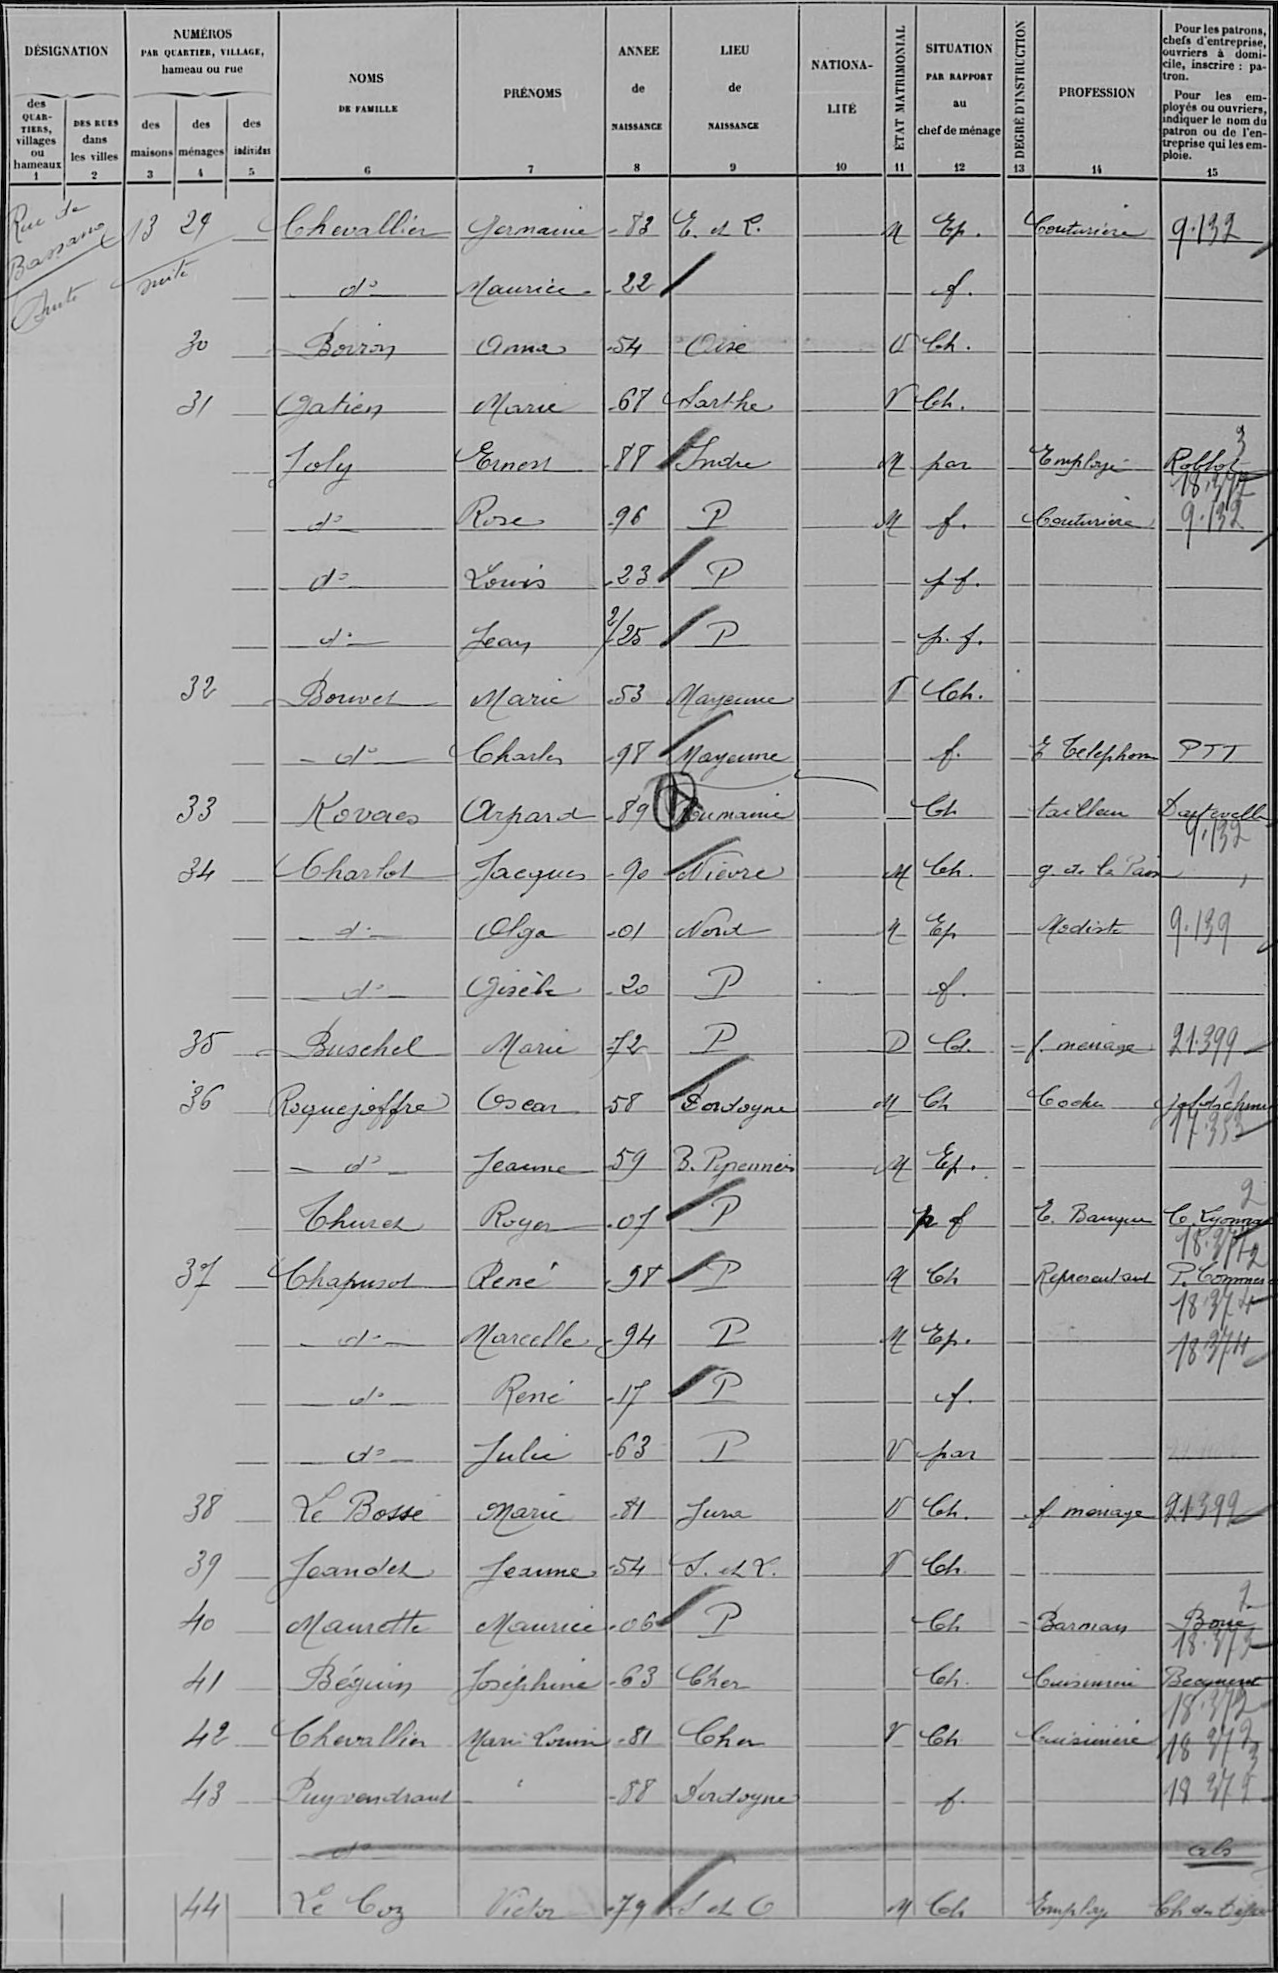Chevallier/Germaine/83/E et L /¤/M/Ep /¤/Couturiere/9132
d°/Maurice/22/¤/¤/¤/f/¤/¤/¤
Boiron/Annie/54/Oise/¤/V/Ch /¤/¤/¤
Gatien/Marie/67/Sarthe/¤/V/Ch /¤/¤/¤
Joly/Ernest/88/Indre/¤/M/par/¤/Employé/Roblot!18377
d°/Rose/96/P/¤/M/f /¤/couturiere/9132
d°/Louis/23/P/¤/¤/f f /¤/¤/¤
d°/Jean/25/P/¤/¤/f f /¤/¤/¤
Bouver/Marie/53/Mayenne/¤/V/Ch /¤/¤/¤
d°/Charles/98/Mayenne/¤/¤/f /¤/E Telephone/PTT
Kovacs/Arpard/89/Roumanie/¤/¤/Ch/¤/tailleur/Dastevelle!9132
Charlot/Jacques/90/Nièvre/¤/M/Ch /¤/g d la Paix/¤
d°/Olga/01/Nord/¤/M/Ep/¤/modiste/9139
d°/Gisèle/20/P/¤/¤/f/¤/¤/¤
Buschel/Marie/72/P/¤/D/Ch /¤/f ménage/21399
Roquejoffre/Oscar/58/Dordogne/¤/M/Ch/¤/Cocher/Joldocheur!17353
d°/Jeanne/59/B Pyrennés/¤/M/Ep /¤/¤/¤
Thurez/Roger/07/P/¤/¤/p f/¤/E Banque/C Lyonnais!18374
Chapusol/René/98/P/¤/M/Ch/¤/Representant/P Commerce!18374
d°/Marcelle/94/P/¤/M/Ep /¤/¤/18374
d°/René/17/P/¤/¤/f /¤/¤/¤
d°/Julie/63/P/¤/V/par/¤/¤/¤
Le Bosse/Marie/84/Jura/¤/V/Ch /¤/f menage/21399
Jeandez/Jeanne/54/I et V/¤/V/Ch/¤/¤/¤
Maurette/Maurice/06/P/¤/¤/Ch/¤/Barman/Boué!18373
Béguin/Joséphine/63/Cher/¤/¤/Ch /¤/Cuisinière/Becquerot!18372
Chevallier/Marie Louise/81/Cher/¤/V/Ch /¤/Cuisinière/18372
Puyvendrant/¤/88/Dordogne/¤/¤/f/¤/¤/18372
d°/¤/¤/¤/¤/¤/¤/¤/¤/abs
Le Coz/Victor/79/S et O/¤/M/Ch/¤/Employé/Ch du Dafeur
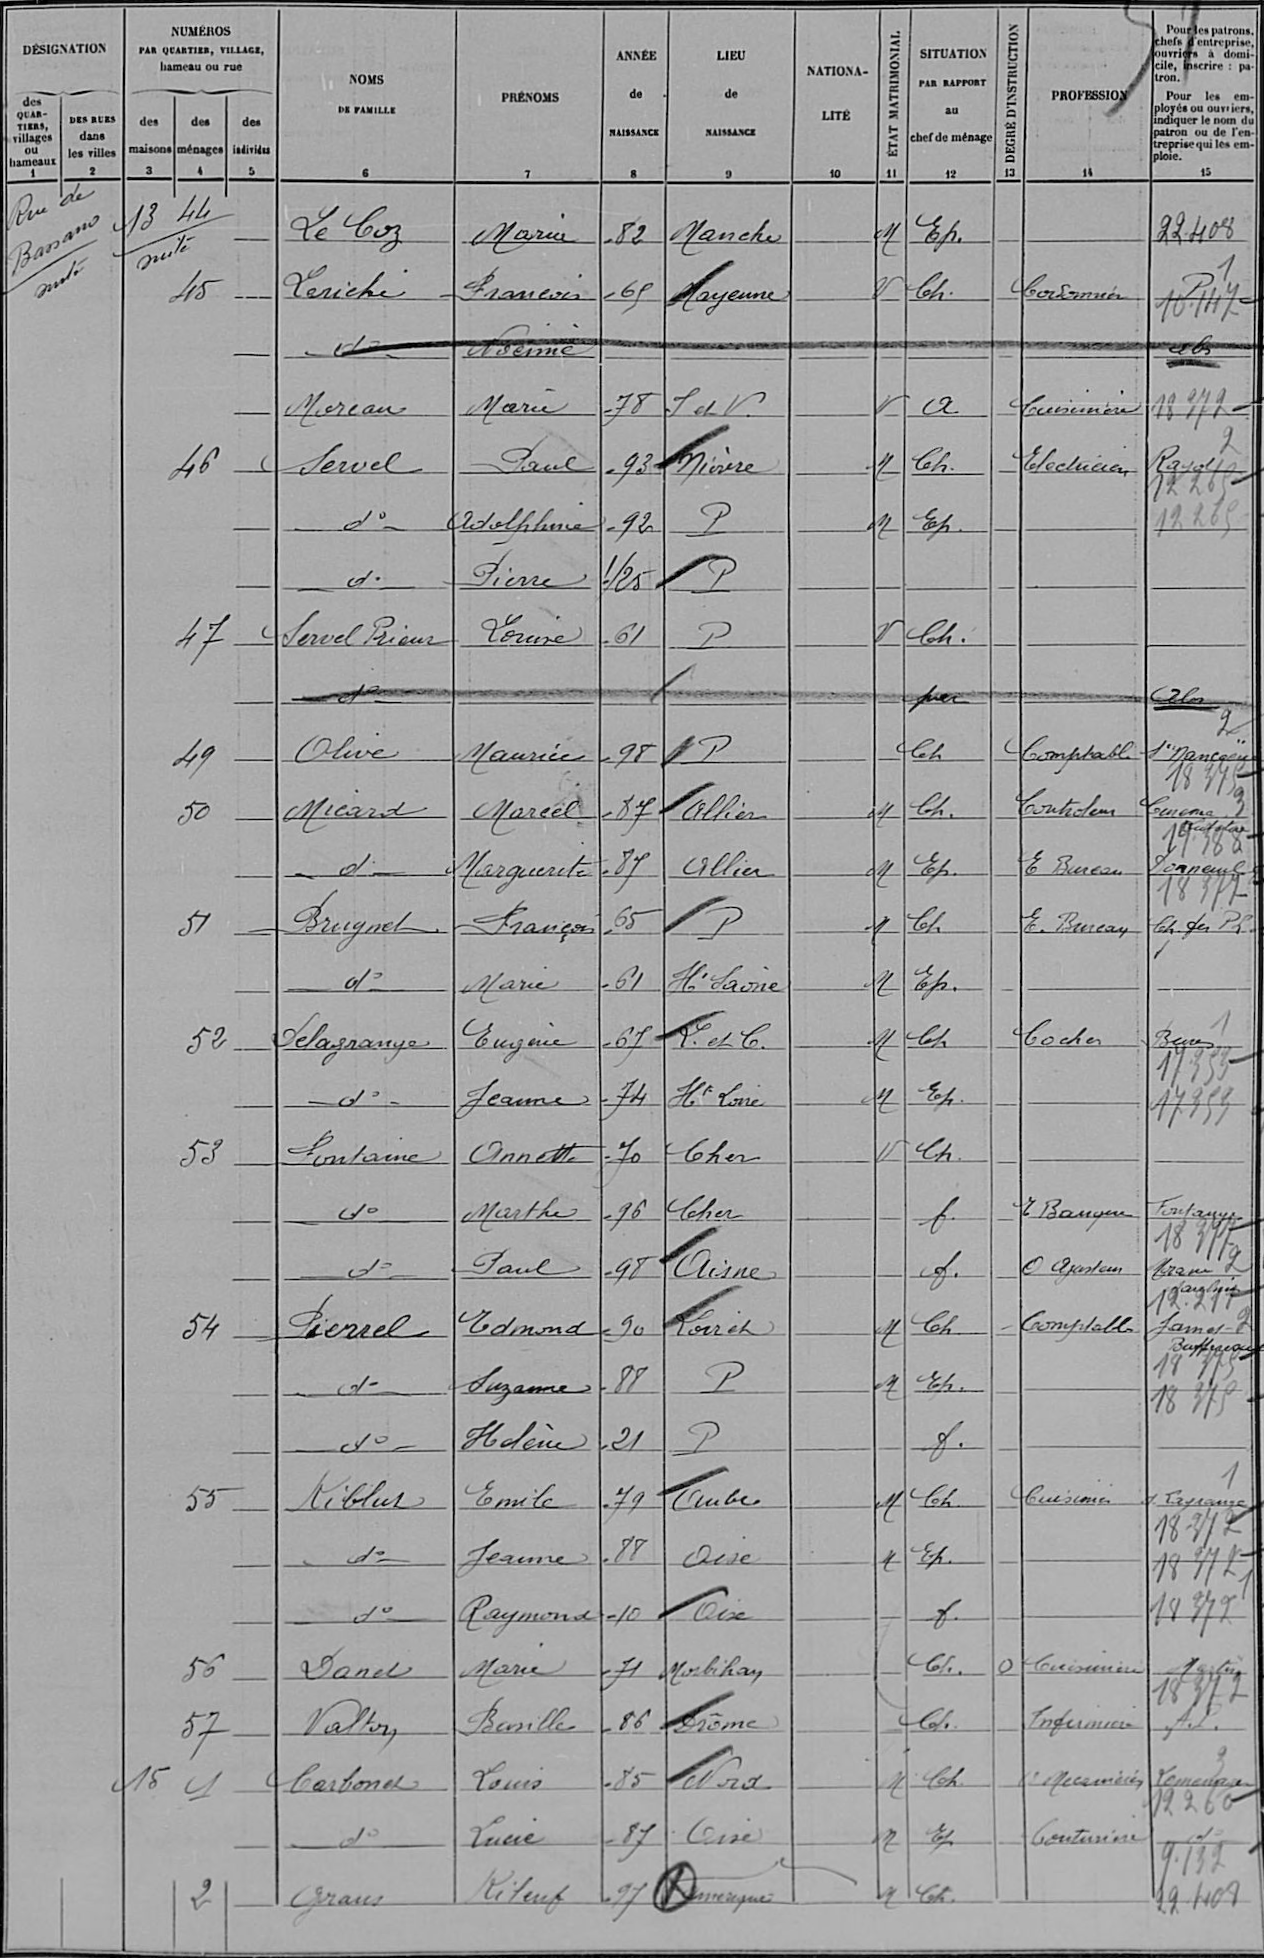Le Coz/Maria/82/Manche/¤/M/Ep /¤/¤/22408
Leriche/Francois/65/Mayenne/¤/V/Ch/¤/Cordonnier/10147
d°/Noémie/¤/¤/¤/¤/¤/¤/¤/abs
Moreau/Marie/78/I et V/¤/V/A/¤/Cuisinière/18372
Servel/Paul/93/Nièvre/¤/M/Ch/¤/Electricien/Rayol 12265
d°/Adolphine/92/P/¤/M/Ep /¤/¤/12265
d°/Pierre/25/P/¤/¤/¤/¤/¤/¤
Servel Prieur/Louise/61/P/¤/V/Ch /¤/¤/¤
d°/¤/¤/¤/¤/¤/par/¤/¤/abs
Olive/Maurice/98/P/¤/¤/Ch/¤/Comptable/Dancoeu!18345
Micard/marcel/87/Allier/¤/M/Ch /¤/Controleur/Cerena!19388
d°/Marguerite/87/Allier/¤/M/Ep /¤/E Bureau/Donneur!18377
Breignet/François/65/P/¤/M/Ch/¤/E Bureau/Ch fer Ph
d°/Marie/64/H Saone/¤/M/Ep /¤/¤/¤
Delagrange/Eugénie/65/L et G/¤/M/Ch/¤/Cocher/Bures!17353
d°/Jeanne/74/H Loire/¤/M/Ep /¤/¤/17353
Fontaine/Annette/70/Cher/¤/V/Ch/¤/¤/¤
d°/Marthe/96/Cher/¤/¤/f /¤/E Banque/Fontagui! 18 397
d°/Paul/98/Aisne/¤/¤/f /¤/O Ajusteur/Morane!12217
Pierrel/Edmond/90/Loiret/¤/M/Ch/¤/Comptable/Jarnet!Baffereau!18375
d°/Suzanne/88/P/¤/M/Ep /¤/¤/18375
d°/Hélène/21/P/¤/¤/f /¤/¤/¤
Kiblur/Emile/79/Aube/¤/M/Ch /¤/Cuisinier/Cayrauge!18372
d°/Jeanne/88/Oise/¤/M/Ep /¤/¤/18372
d0/Raymond/10/Oise/¤/¤/f /¤/¤/18372
Danel/Marie/71/Morbihan/¤/¤/Ch /O/Cuisinière/Martin!18372
Valton/Basille/86/Drôme/¤/¤/Ch/¤/Infirmière/AP
Carboner/Louis/85/Nord/¤/M/Ch /¤/Mécanicien/Lemenage!12260
d°/Lucie/87/Oise/¤/M/Ep/¤/Couturiere/9132
Graus/Kiteuf/97/Auvergne/¤/M/Ch /¤/¤/22408